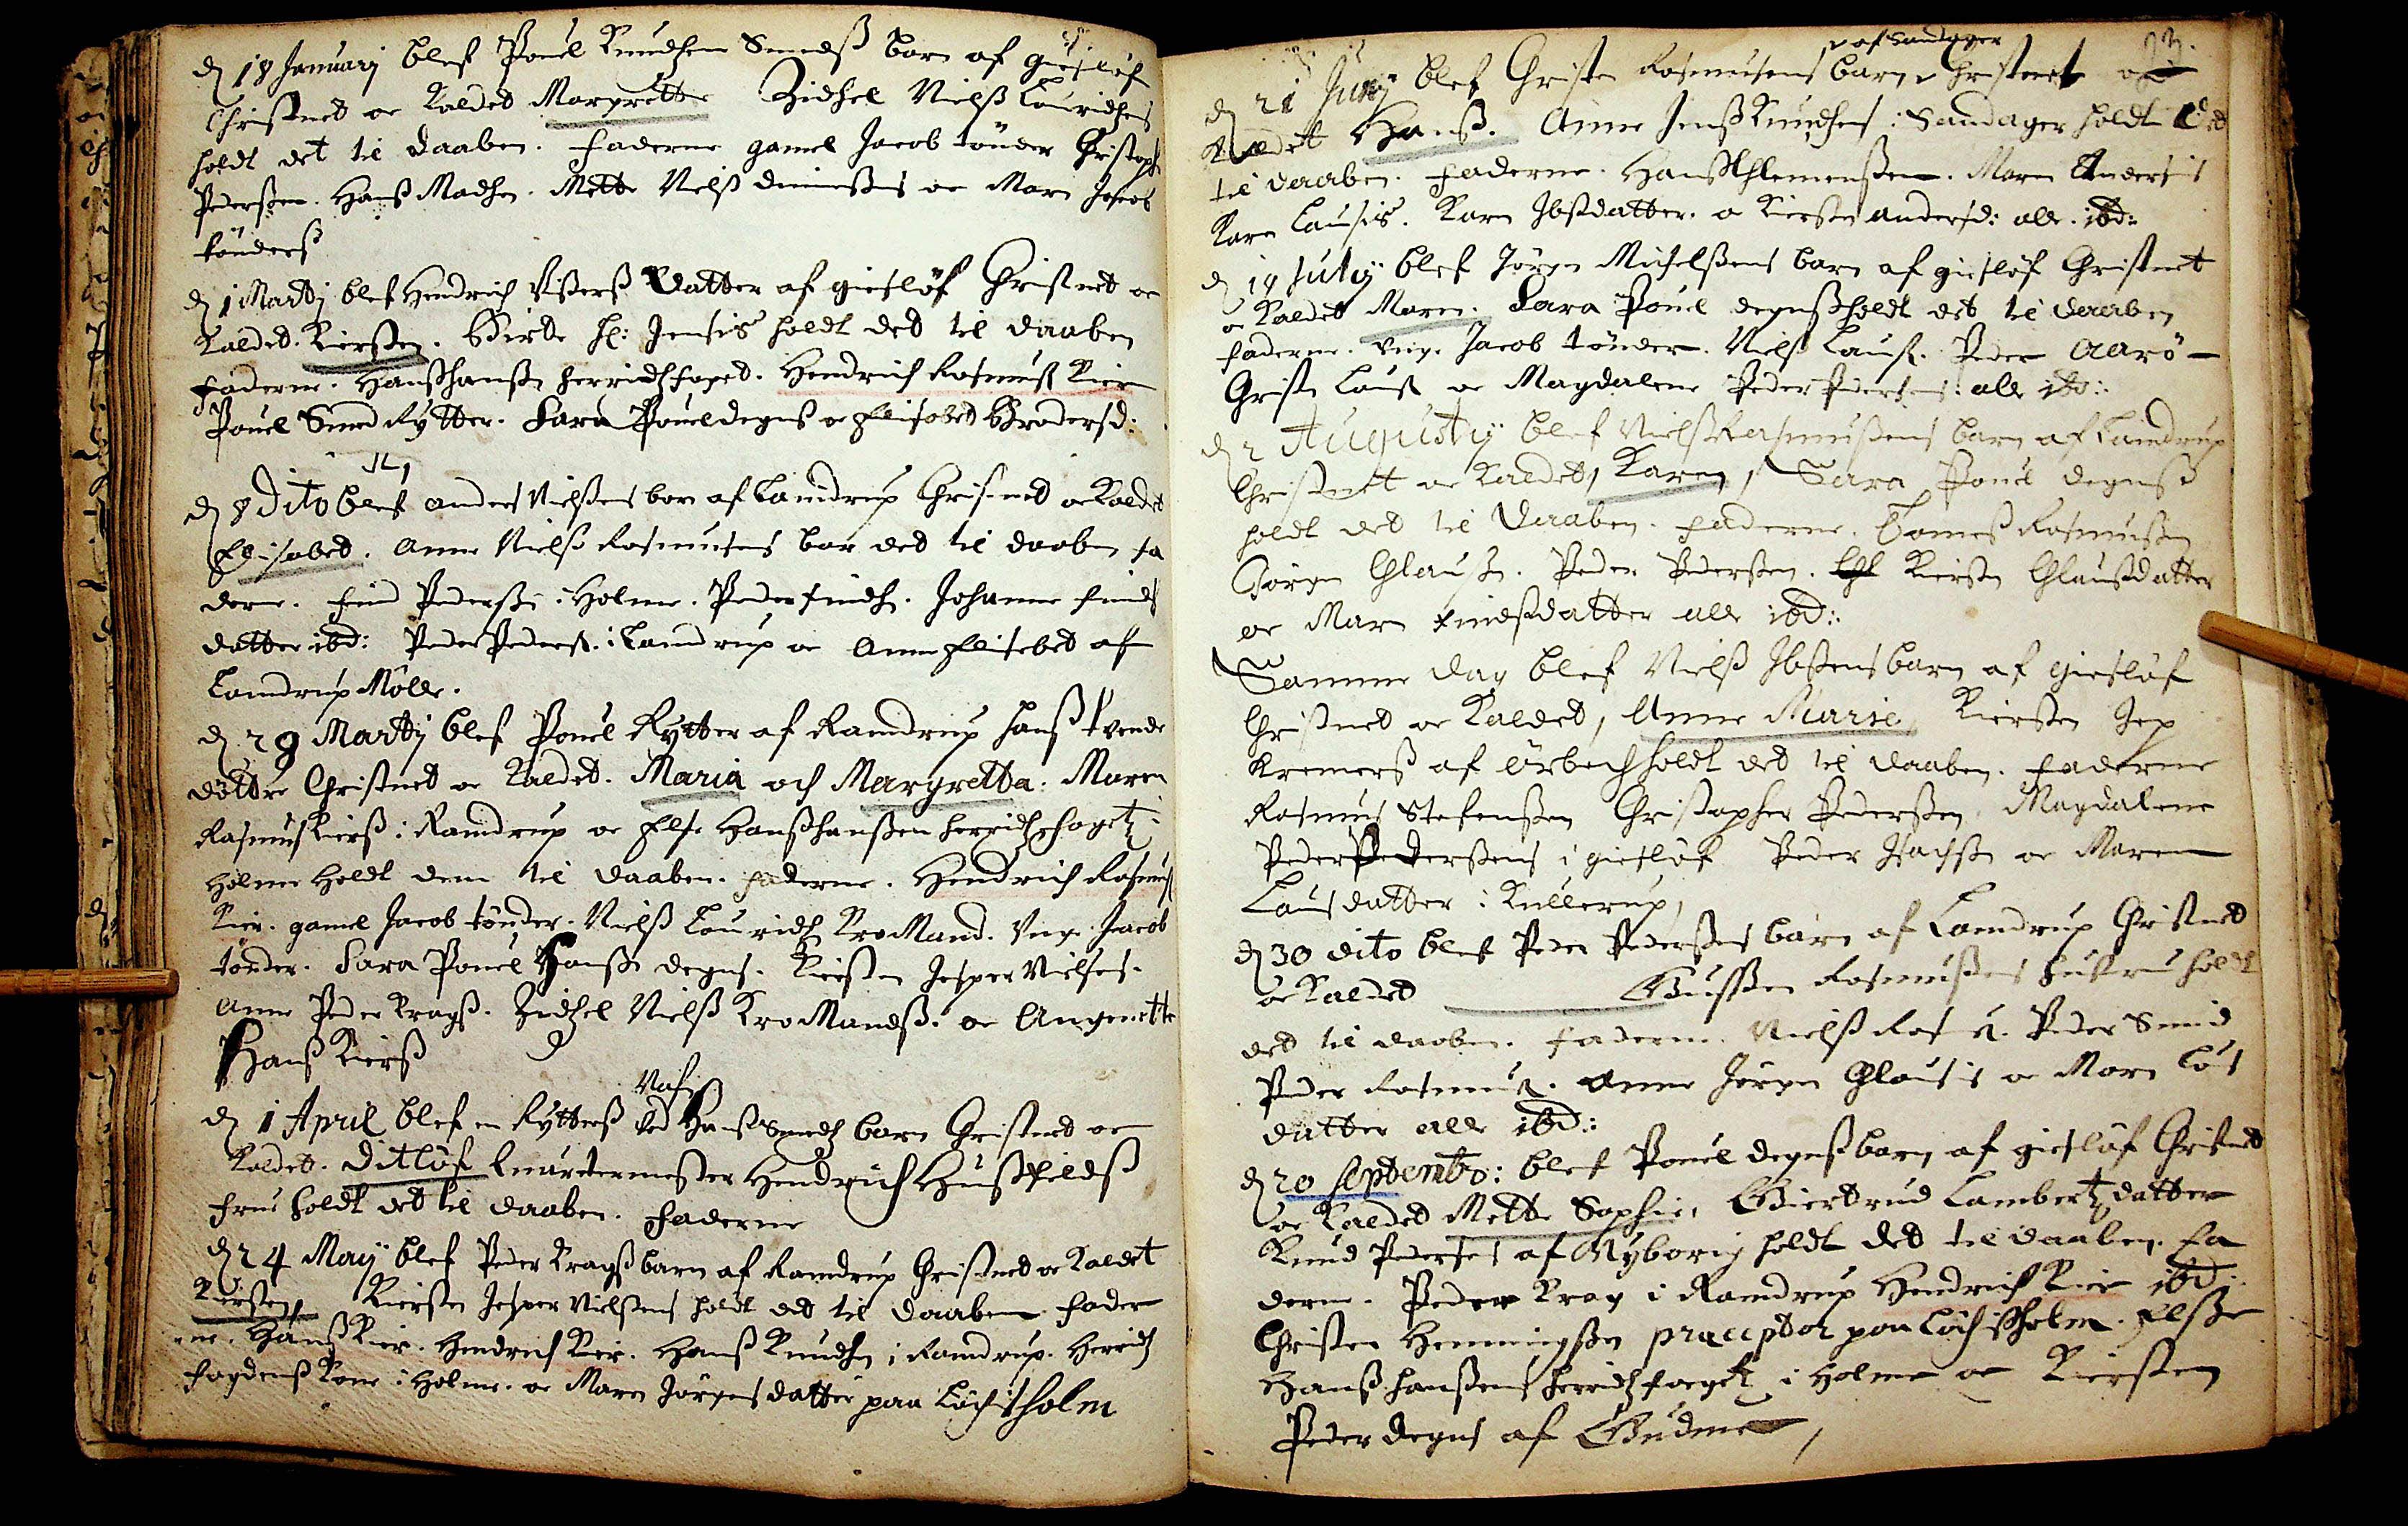d 19 Jnuarij blef Pouel Knudtzen Smedss barn aff Giesløf
Christnet oc kaldet Margrette Zidhel Nielss Lauridtzens
holdt det til daaben. Faderne gamel Jacob tønder Christoh  
Pederssen. Hans Madsen. Mette Niels dinnessens oc Maren Jacob
tønderss
d 1 Martij blef Hendrich visterss Datter af Giesløf Christnet oc
Kaldet Kiersten. Birte Hl. Jensis holdt det til daaben.
Faderne. Hans Hanssen Herridtzfoget. Hendrich Rasmus Kier
Pouel Smed Rytter: Sara Pouel degns oc Elisabet Brodersd:
d 8 Dito blef Anders Nielssens bar af Lamdrup Christnet oc kaldet
Elisabet. Anne Niels Rasmusens bar det til daaben fa
derne. Find Pederssen i Holme. Peder Finds. Johanne Finds
datter ibd: Peder Peders i Lamdrup oc Anne Elisebet af
Landrup Mølle.
d 28 Martij blef Pouel Rytter af Ramdrup Hanss tvende
døttre Christnet oc Lredod: Maria och Margretta. Maren
Rasmus Kierss i Ramdrup oc Else Hanss Hanssen Herridzefoget
Holme holdt der tel Daaben. Faderne. Hendrich Rasmus
KPier: gamel Jacob Tørder: Nielss Lauridtz Kro Mand. vnge Jacob
tønderr. Sara Pouel Hanss Degns: Kiersten Jesper Nielsens
Anne Peder Kragss. Zidtzel Nielss Kro Mandss oc Angenette
Hans Kierss
Nafn
d 1 April blef en Rytters ved Hanss smedtz barn Christed oc
kaldet. Ditløf Quartermester Hendrich Husteldss 
Fru holdt det til Daaben. Faderne
d 24 Maij blef Peder Kragss barn af Ramdrup Christed oc kaldet
Kiersten, Kiersten Jesper Nielssens holdt det til Daaben. Fader
= ne Hans Kier Hendrich Kier. Hanss Knudtzen i Ramdrup. Herridtz
fogdenss Kone i Holme oc Maren Jørgensdatter paa Løchisholm
23.
v af Sandager
D. d1 Julnj blef Christen Rosmusens barn v Christenet och
kaldet Hanss. Anne Jenss Knudtzens: Sandager holdt det
til daaben. Faderee. Hanss Clemenssen. Maren Andersis
Karn Lausis. Karen Ibsdaatter, oc Kiersten Andersd: alle. ibd:.
d 19 Julij blef Jørgen Michelsens barn af giesløf Christnet
ocr kaldet Maren. Sara Pouel degnss holdt det til Daaben
Faderne. vnge. Jacob Tønder. Niels Laus, Peder Aarø
Christen Laus oc Magdalene Peder Pdersens alle ibd:.
d 2 Augustij blef Niels Rasmusens barn af Lamdrup
Christnet oc kaldet Karen Saran Pouel degnss
holdt det til Daaben. Faderne Tomess Rasmussen
SJøren Chlausen. Peder Pedersen ## Kiersten Chlaussdatter
oc Marn Findssdatter alle ibd:.
Samme dag blef Nielss Ibsens barn af Giesløf
Christnet oc kaldet Anne Marie Kiersten Jep
Kremers af Ørbec holdt det til daaben. Fadfrene
Rasmus Stefenssen Christopher Pederssen Magdalene
Peders Pederssens i Giesløf, Peder Jachse oc Maren
Lasdatter i Krllerup
d 30 dato blef Peder Isachssen barn af Lamdrup Christnet
oc kaldet
Gustssen Rasmussens Hustru holdt
det til daaben. Faderne Niels Rasmus. Peder Smid
Peder KRasmus. Aene Jergen Chlausis oc Maren Laus
datter ale ibd:.
d 20 Septembr: blef Pouel Degnss barn af giesløf Christenet
oc kaldet Mette Sophie Giertrud Lambertz datter
Knud Pedersens af Nyborig holdt det til daaben, Fa
derne. Peder Krap i Ramdrup Hendrich Kier ibd
Christen Henningssen praccptor poa Løchissholm Elsse
Hanss Hanssens Herridtzfoeget i Holme oc Kiersten
Peder Degns af Gudme,
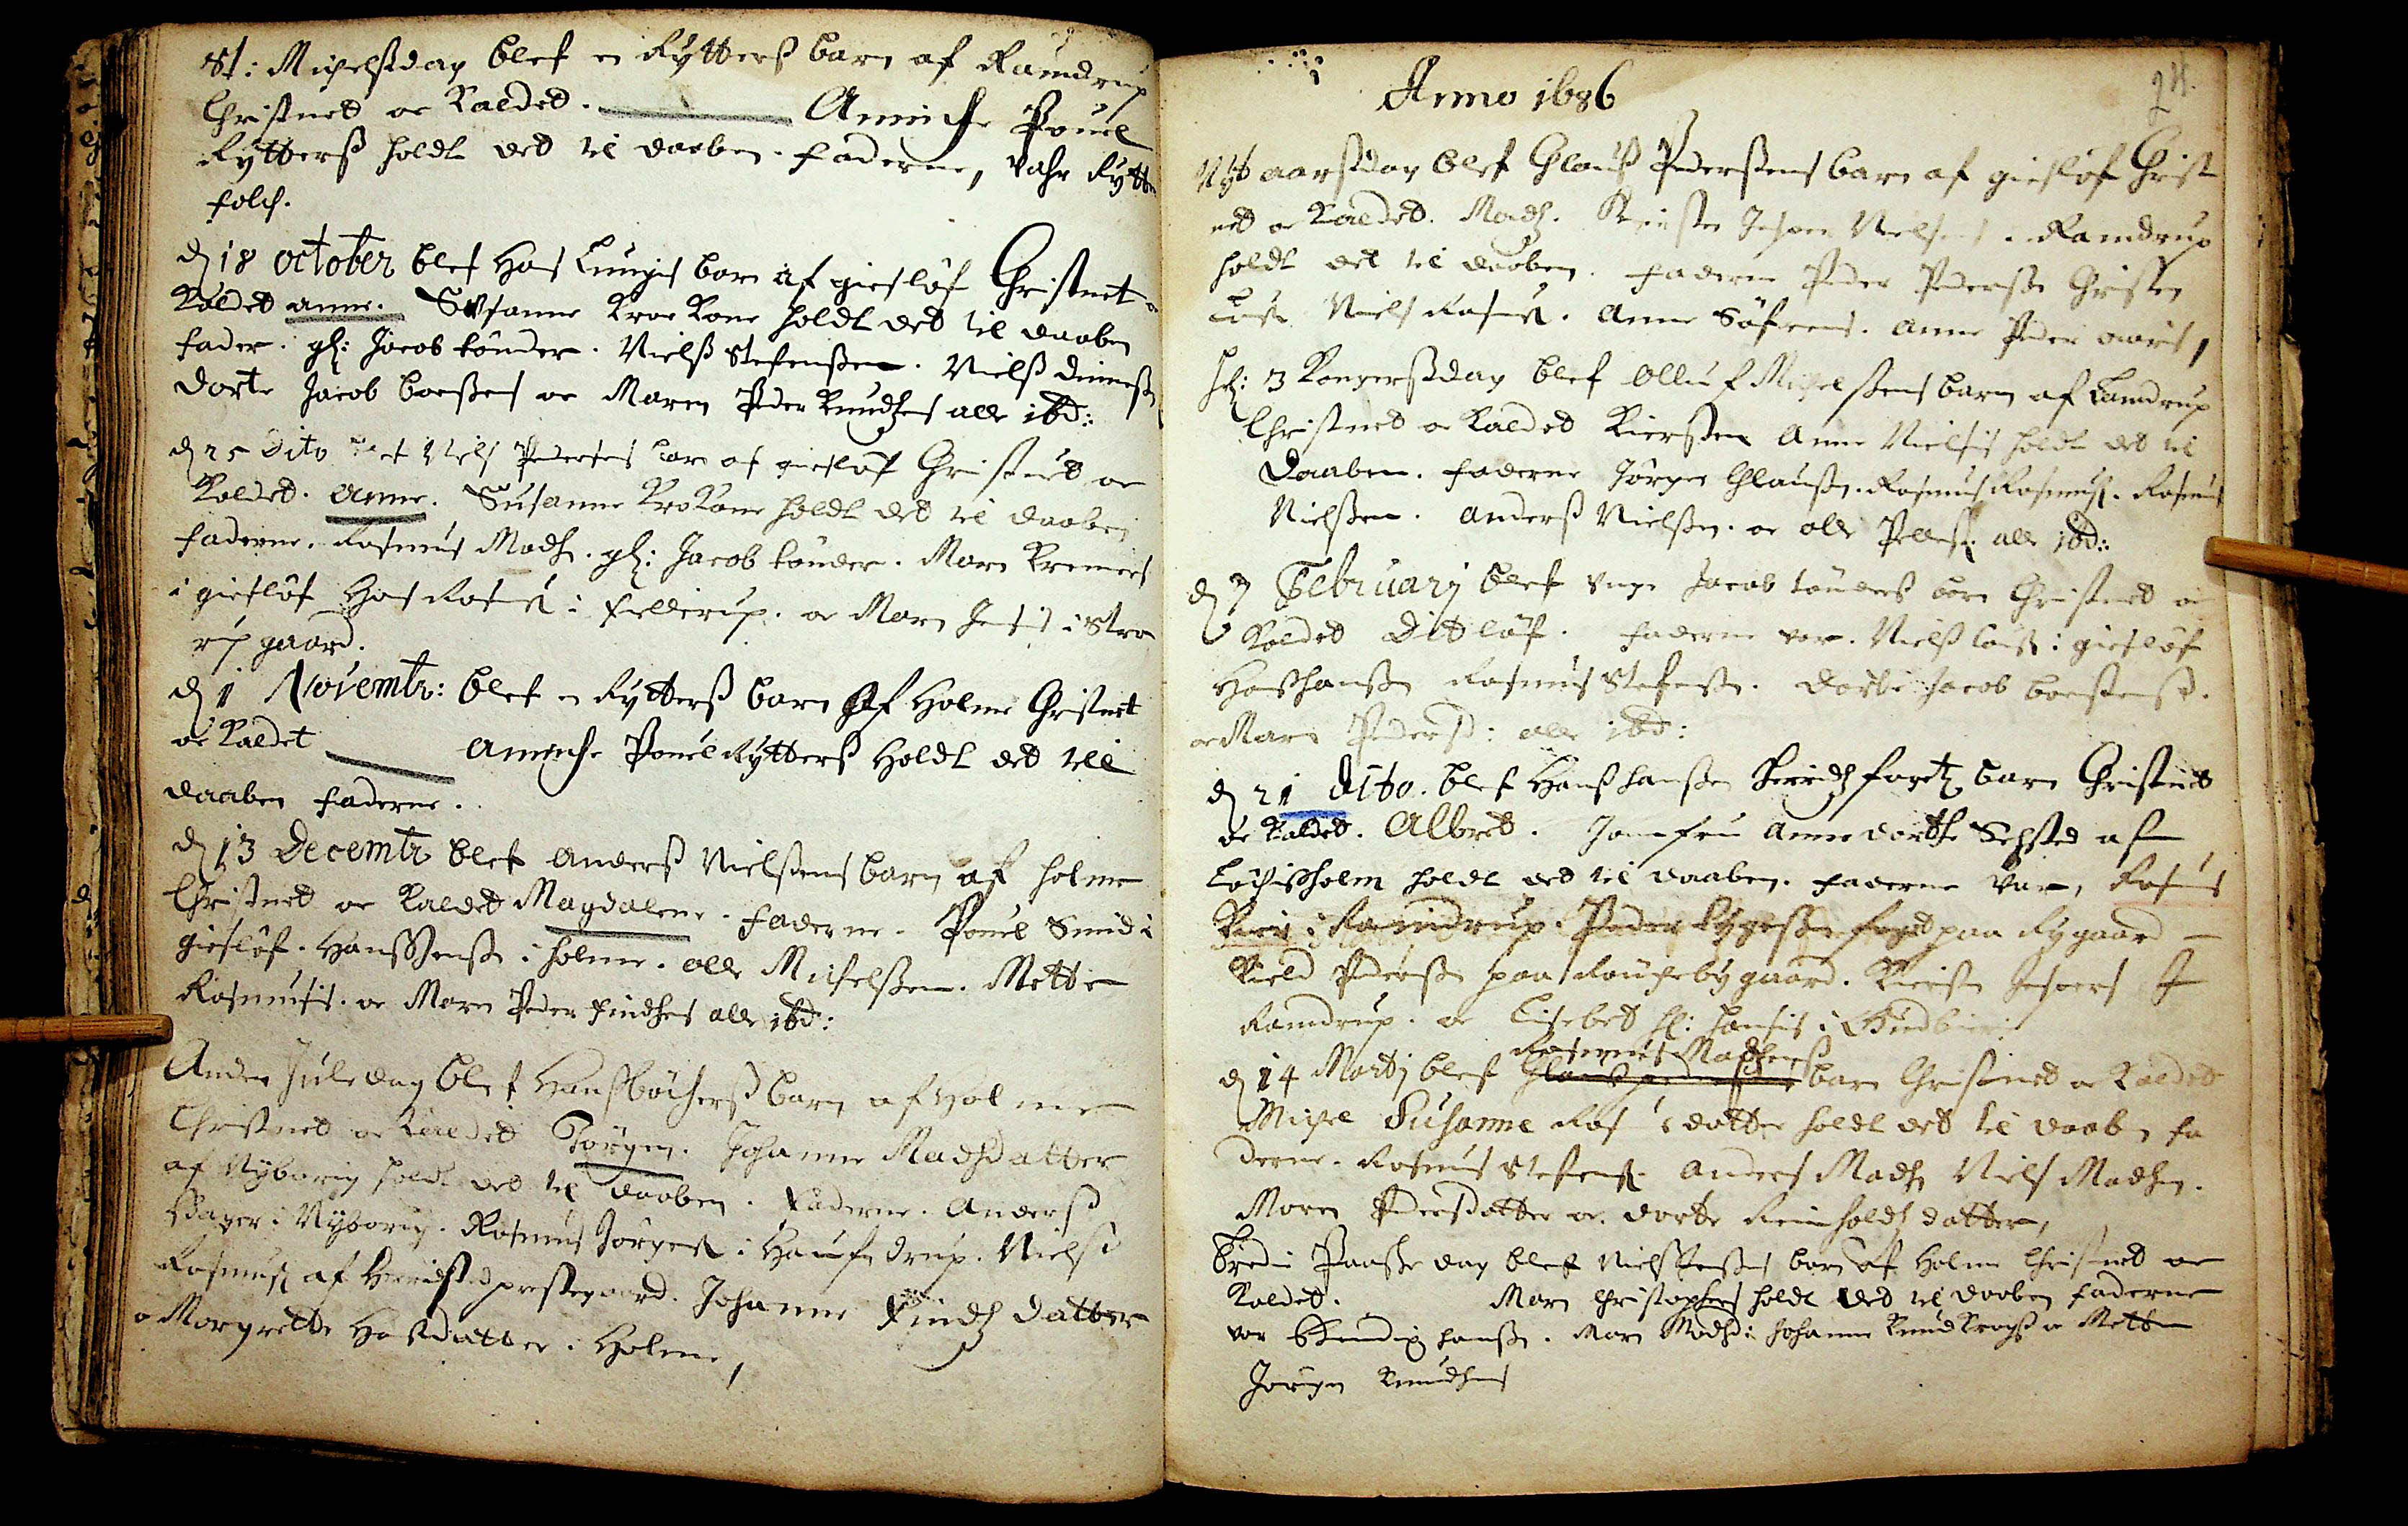St: Michelcsdag bleff en Rytters barn aff Ramdrup
Christned oc kaldet
Anniche Pouel
Rytters holdt det til daaben. Faderne, Vafr Rytter
folch.
d 18 October blef Hans Lungis barn af giesløf Christnet oc
kaldet Anne. Svsanne Kroe Kone holdt det til daaben
fader: gl: Jacob Tønder. Nielss Stefenssens. Niels Dinness
Dorte Jacob Boesen oc Maren Peder Knudtzens alle ibd:.
d 25 Dito blef Niels Pedersens barn af giesløf Christnet oc
koldet. Anne. Susanne Kro Kone holdt det til daaben
Faderne Rasmus Mads gl: Jacob tønder. Maren Kremers
i giesløf Hans Rasmus i Fiellerup oc Maren Jensis i Stro
rup gaard.
d 1 Novembr: blef en Rytters barn af Holme Christnet
oc kaldet
Anniche Pouel Rytters Holdt det tll
daaben Faderne.
d 13 Decembr blef Anderss Nielsens barn af holme
Christnet oc kaldet Magdalen. Faderne Pouel Smed i
Giesløf. Hanss Jenss i holme. Olle Michelssen. Mette
Rasmusis oc Maren Peder Findses alle ibd:
Anden Juledag blef Hans Bøchers barn af Holme
Christnet oc kaldet Jørgen. Johanne Madsdatter
af Nyborig holdt det til daaben. Faderne. Anderss
Bager i Nyborig Rasmus Jørgens i Haufndrup Nielss
Rasmus af Herridsted prestegaard. Johanne Findtz datter
o Margrette Hanssdatter i Holme,
24.
Anno 1686
Nyt aarsdag blef Chlaus Pederssens barn af Giesløf Christ
net oc Kaldet Mandtz. Kirsten Jesper Nielsens i Ramdrup
holdt det til daaben. Faderen Peder Pederss Christen
Lauss Niels Rasmus. Anne Søfrens. Anne Peder Aaris,
Hel: 3 Kongerssdag blef Olluf Michelssens barn af Landrup
Christnet oc kaldet Kiersten Anne Nielsis høidt det til
Daaben. Faderne Jørgen Chlaussen, Rasmus Rasmuss. Rasmus
Nielssen. Anderss Nielssen oc Olle Pelles alle ibd:.
d 7 Februarij blef vnge Jacob tønderss barn Christnet oc
kaldet Ditløf. Faderne var Nielss Lauss i Giesløf
Hans Hanssen Rasmus Stefensen. Dorte Jacob Boessenss
oc Maren Pedersd: alle ibd:
d 21 dito. blef Hanss Hanssen Herridtzfogetz barn Christenet
de kaldet. Albret. Jomfru Ane dorthe Sehsted af
Løchisholm holdt det til daaben. Faderene var Rasmus
Kier i Ramdrup. Peder Tygessen foget paa Ryygaard
Kield Pederssen paa Rouchebygaard. Kiersten Jespers I
Ramdrup. oc Eisebet Hl: Hansis i Gudbierg
Rasmus Madtzenss
d 14 Martij blef Chlaus pedersens i barn Christned oc kaldet
Michel Susanne Rasmusdatter holdt det til daab; Fa
derne. Rasmus Stefenss. Anders Mads Niels Madsen.
Maren Pedersdatter or. Dorte Reinholdtz datter,
Tredie Paaske dag bleff Nielss Jenssens børn af Holme Christnet oc
kaldet.
Marn Christophers holdt det til daaben. Faderne
var Bendix Hanssen. Marn Madsd: Johanne Knud Kragss oc Mette
Jørgen Knudsens